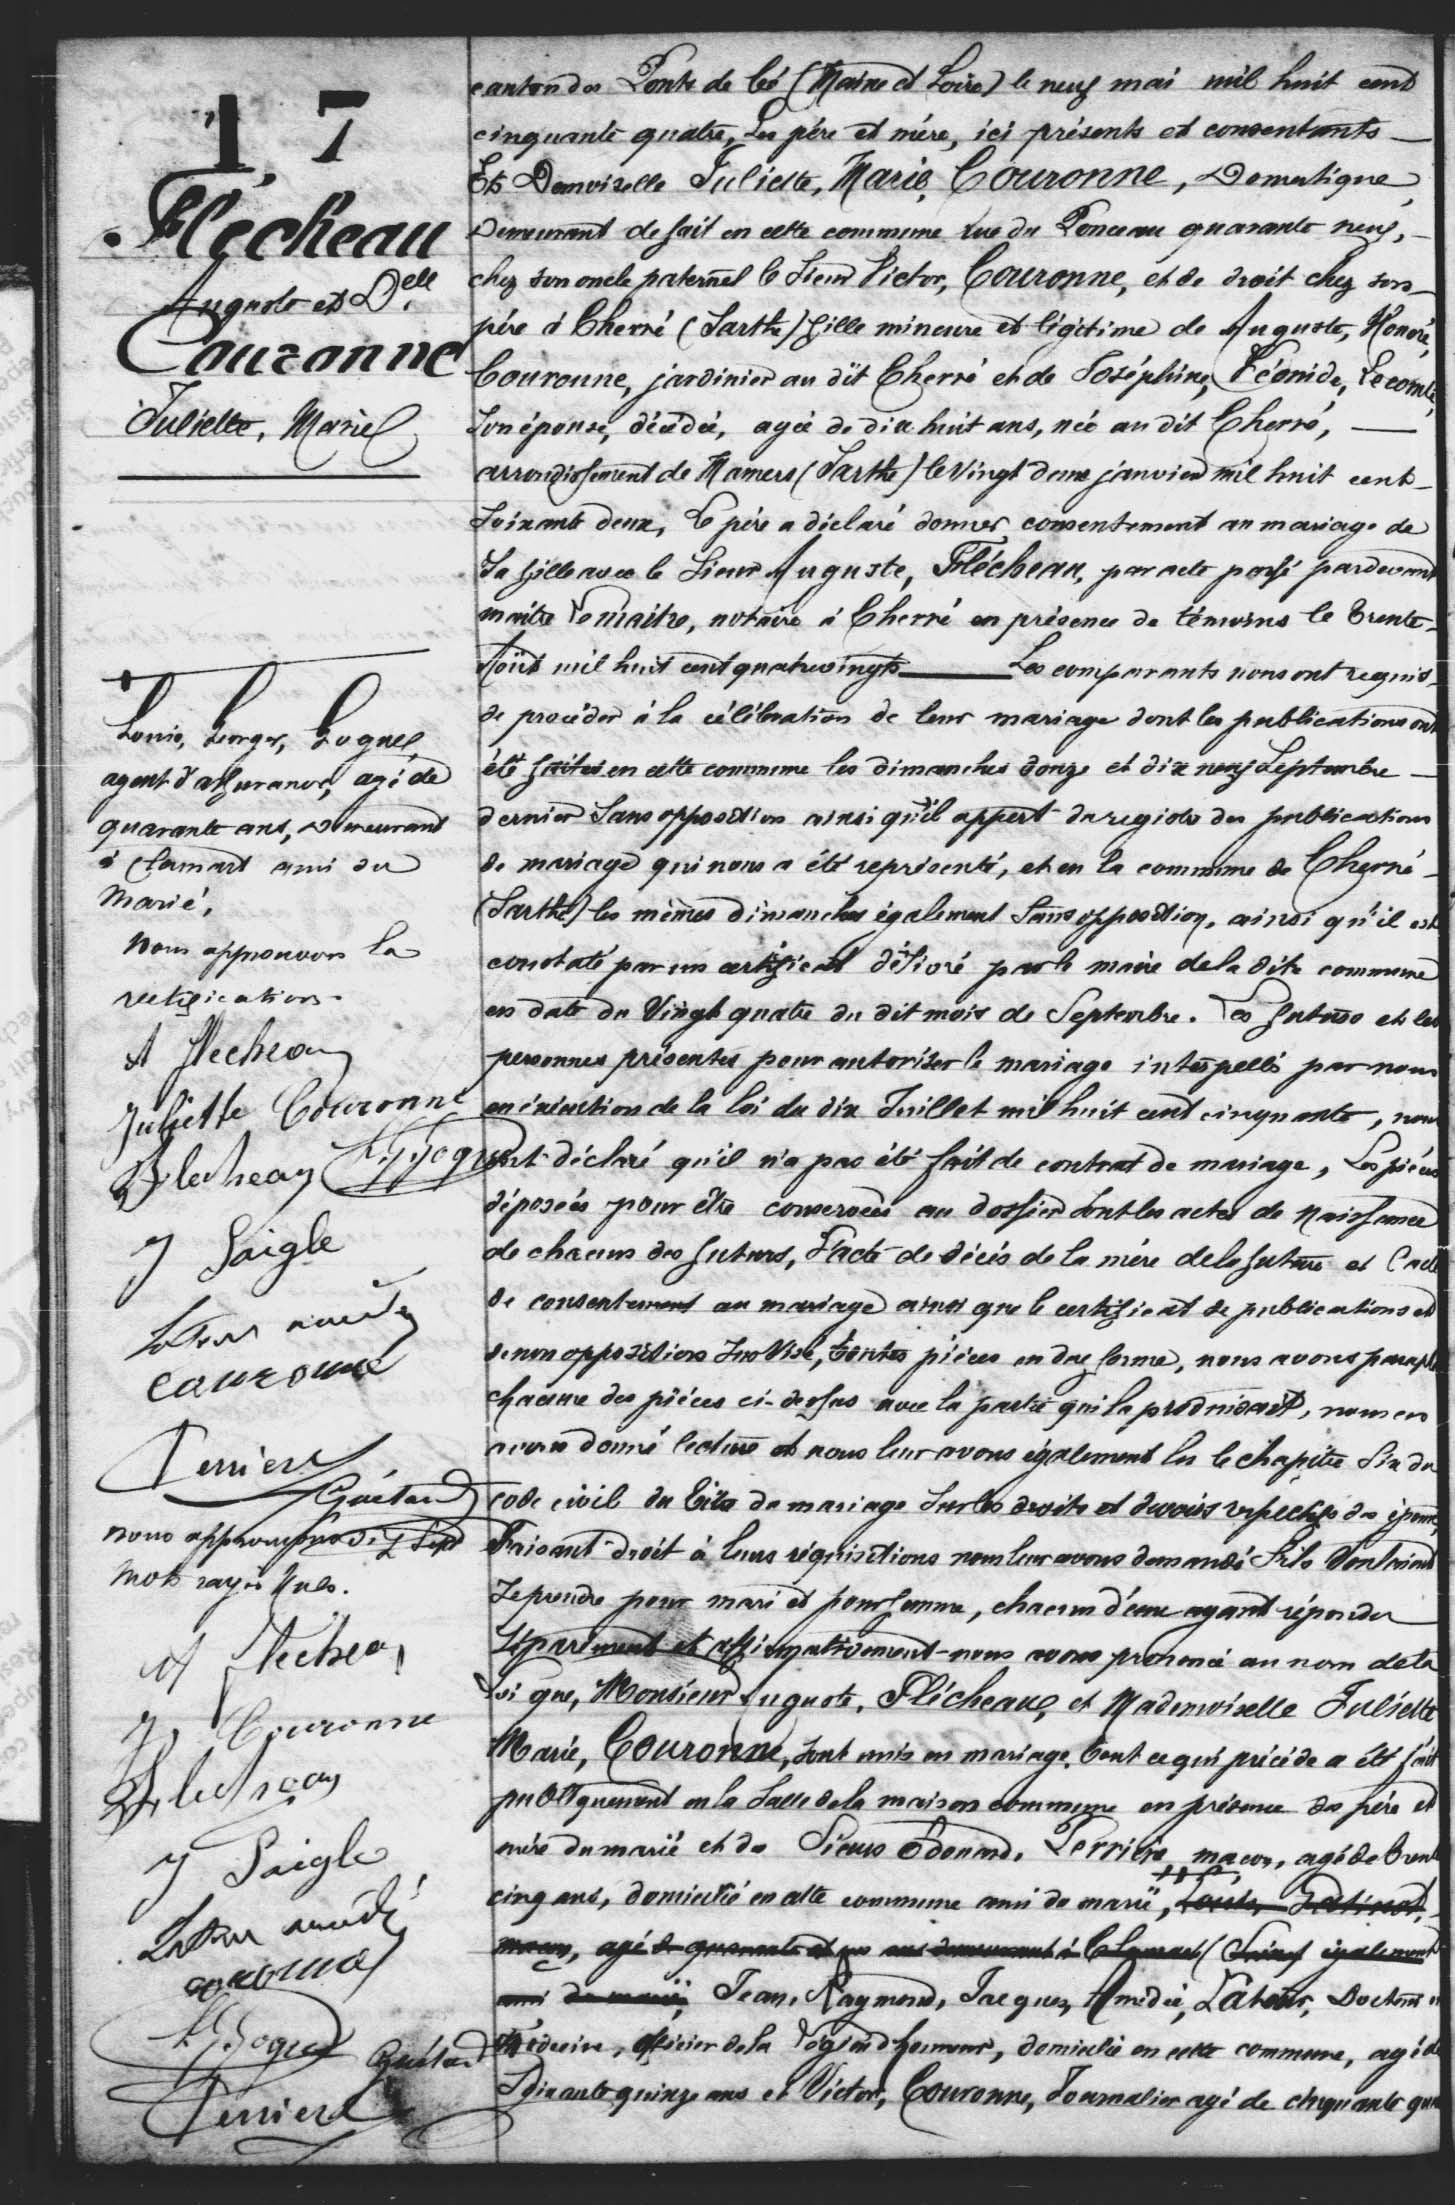17
Flécheau
Auguste et Dell
Couronne
Juliette, Marie
canton des Ponts de Cé (Maine et Loire) le neuf mai mil huit cent
cinquante quatre, les père et mère, ici présents et consentants -
Et Demoiselle Juliette, Marie, Couronne, Domestique,
Demeurant de fait en cette commune rue du Ponceau quarante-neuf,-
chez son oncle paternel le Sieur Victor, Couronne, et de droit chez son
père à Cherné (Sarthe) fille mineure et légitime de Auguste, Honoré,
Couronne, jardinier au dït Cherné et de Joséphine, Léonide, Lecomte,
son épouse, décédée, agée de dix huit ans, née au dit Cherné, -
arrondissement de Mamers (Sarthe) le vingt deux janvier mil huit cent-
soixante deux. Le père a déclaré donner consentement au mariage de
sa fille avec le Sieur Auguste, Flécheau, par acte passé pardevant
son maitre. Le maitre, notaire à Cherné en présence de témoins le trente
Août mil huit cent quatre vingts-Les comparants nous ont requis
de procéder à la célébration de leur mariage dont les publications ont
été faites en cette commune les dimanche douze et dix neuf Septembre -
dernier sans opposition ainsi qu'il appert le registre des publications
de mariage qui nous a été représenté, et en la commune de Cherné-
(Sarthe) les mêmes dimanches également sans opposition, ainsi qu'il est
constaté par un certificat délivré par le maire de la dite commune
en date du vingt quatre du dit mois de Septembre. Les futurs et les
personnes présentées pour autoriser le mariage interpellés par nous
en réactions de la Loi du dix Juillet mil huit cent cinquante, nous
ont déclaré qu'il n'a pas été fait de contrat de mariage, Les pièces
déposées pour être conservées au dossier dont les acte de naissance
de chacun des futurs, d'acte de décès de la mère de la future et acte
de consentement au mariage ainsi que le certificat de publications et
de non opposition Jrovise, toutes pièces en due forme, nous avons paraphé
chacune des pièce ci-dessus avec la partie qui la produisait, nous en
avons donné lecture et nous leur avons également lu le chapitre six du
code civil du livre du mariage sur les droits et devoirs respectifs des époux,
faisant droit à leurs réquisitions nous leur avons demandé s'ils voulaient
se prendre pour mari et pour femme, chacun d'eux ayant répondu
séparément et affirmativement nous sommes prononcé au nom de la
loi que, Monsieur Auguste, Flécheaux et Mademoiselle Juliette
Marie, Couronne, sont unis en mariage. Tout ce qui précède a été fait
publiquement en la salle de la maison commune en présence des père et
mère du mariré et des sieurs ordonné. Perrière, maçon, agé de trente
cinq ans, domicilié en cette commune ami du marié, Louise Antoine
Marie: agé de quarante et un ans, demeurant à la Colombe (Seine) signalement
ami du marié; Jean Raymond, Jacques Amédée, Latour, Docteur en
médecine, Officier de la Légion d'honneur, domicilié en cette commune, agé de
Soixante-quinze ans et Victor, Couronne, journalier, agé de cinquante ans
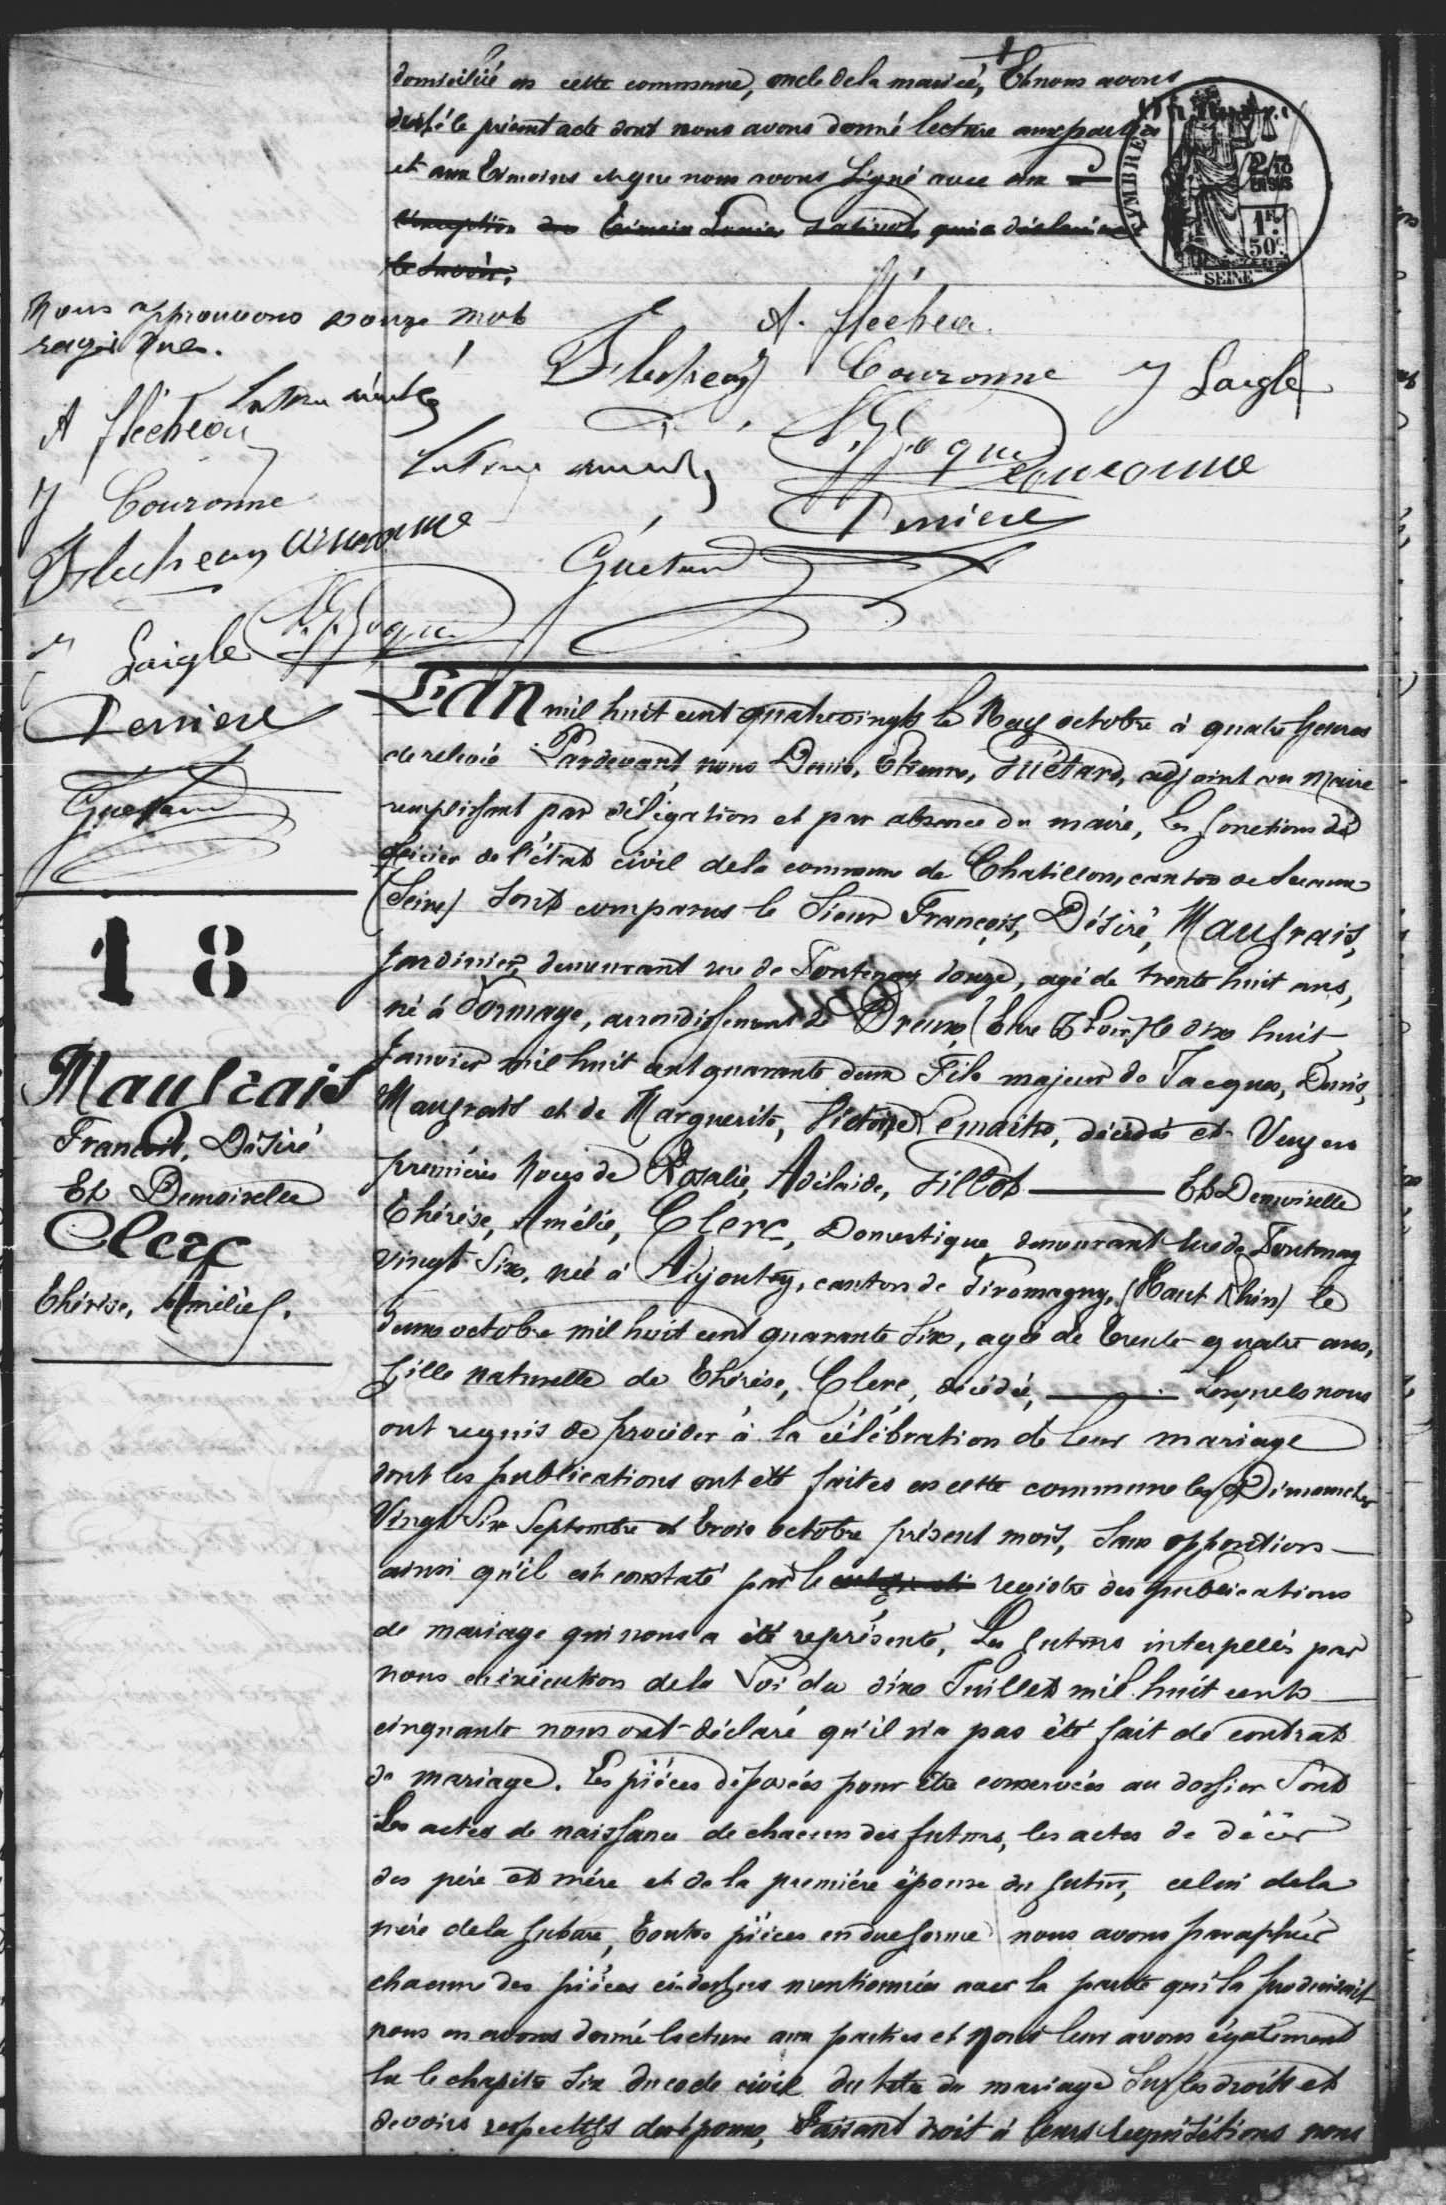domicilié en cette commune, oncle de la mariée, Et nous avons
signé le présent acte dont nous avons donné lecture aux parents
et aux témoins et que nous avons signé avec eux
18
Maufrais
François, Désiré
Et Demoiselle
Clerc
Thérèse, Amélie
L'an mil huit cent quatre vingt le neuf octobre à quatre heures
de relevé. Pardevant nous David, Etienne, Guéstard, adjoint au maire
remplissant par délégation et pour absence du maire, les fonctions d'
officier de l'état civil de la commune de Chatillon, canton de Sceaux
(Seine) sont comparus le Sieur François, Désiré, Maufrais,
jardinier demeurant rue de Fontenay douze, agé de trente huit ans,
né à Domange, arrondissement de Dreux (Eure et Loire) dix huit,
Janvier mil huit cent quarante deux fils majeur de Jacques, David,
Maugratt et de Marguerite, Victoire Lemaitre, décédé et veuf en
première noces de Rosalie Adélaide, Fillon -Et Demoiselle
Thérèse, Amélie, Clerc, Domestique, demeurant rue de Sontmag
vingt-six née à Anjoutey, canton de Giromagny (Haut Rhin) le
douze octobre mil huit cent quarante six, agée de trente quatre ans,
fille naturelle de Thérèse, Clerc, décédée, -Lesquels nous
ont requis de procéder à la célébration de leur mariage
dont les publications ont été faites en cette commune les Dimanches
vingt-six septembre et trois octobre, présent mais, sans oppositions-
ainsi qu'ils ont constaté par le registre des publications
de mariage qui nous a été représenté, les futurs interpellés par
nous en exécution de la loi du dix juillet mil huit cent -
cinquante nous ont déclaré qu'il n'a pas été été fait de contrat
de mariage. Les pièces déposées pour être conservées au dossier sont
Les actes de naissance de chacun des futurs, les actes de décès
des père et mère et de la première épouse du futur, celui de la
mère de la future, toutes pièces en due forme nous avons paraphé
chacune des pièces ci dessus mentionnées avec la partie qui la produisait
nous en avons donné lecture aux parties et nous leur avons également
lu le chapitre six du code civil du titre du mariage sur les droits et
devoirs respectifs des époux, faisant droit à leurs réquisitions nous